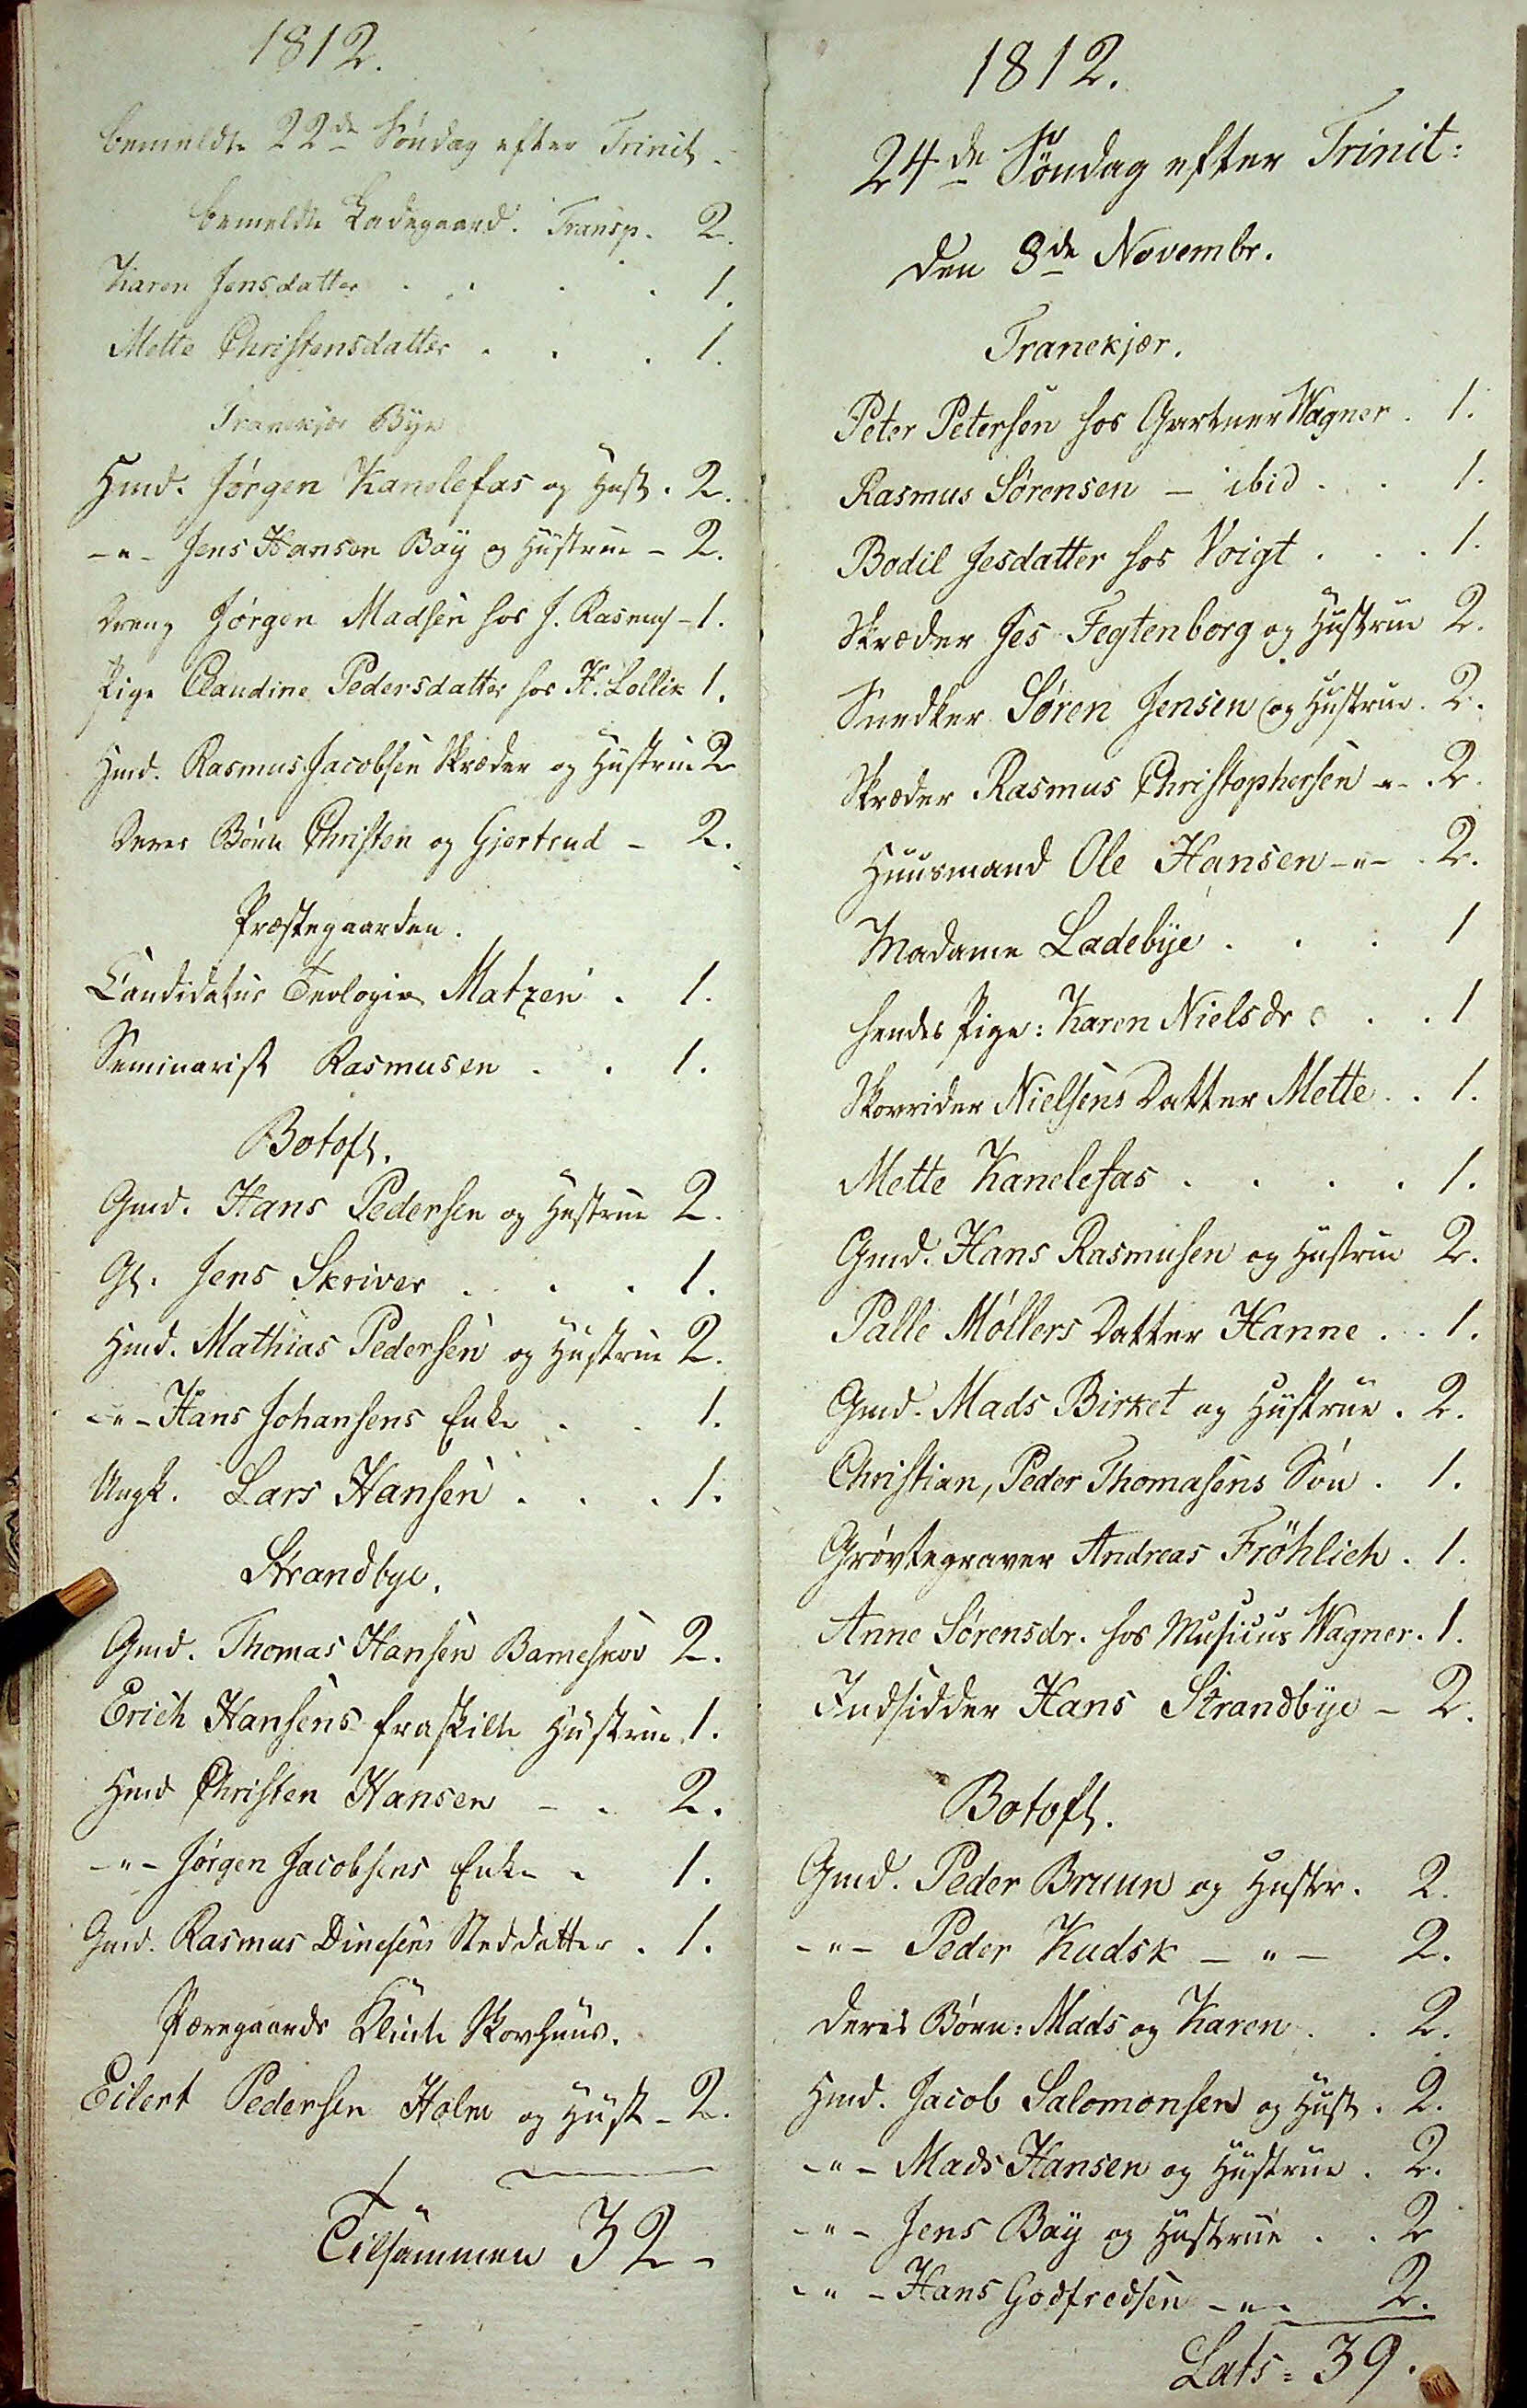1812
bemeldte 22de Søndag efter Trinit.
bemeldte Ladegaard. Transp. 2.
Karen JensDatter . . . . 1.
Mette Christensdatter . . . 1.
Tranekjær Bye
Hmd. Jørgen Kanelefas og Hust . 2.
-"- Jens Hansøn Bay og Hustrue - 2.
Dreng Jørgen Madsen hos J Rasmus - 1.
Pige Claudine Pedersdatter hos H. Lollik 1.
Hmd. Rasmus Jacobsen Skræder og Hustrue 2
deres Børn Christen og Gjertrud - 2.
Præstegaarden.
Candidatus Teologie## Matzen . 1.
Seminarist Rasmusen . . 1.
Botoft.
Gmd. Hans Pedersen og Hustrue 2.
Gl. Jens Skriver . . . 1.
Hmd. Mathias Pedersen og Hustrue 2.
-"- Hans Johansens Enke . . 1.
Ungk. Lars Hansen . . . 1.
Strandbye.
Gmd. Thomas Hansen Bameskov 2.
Erich Hansens fraskilt Hustrue . 1.
Hmd Christen Hansen - . 2.
-"- Jørgen Jacobsens Enke . 1.
Gmd. Rasmus Dinesens Steddatter . 1.
Pæregaards Klint Skovhuus.
Eilert Pedersen Holm og Hust - 2.
Tilsammen 32 -
1812.
24de Søndag efter Trinit:
den 8de Novembr.
Tranekjær.
Peter Petersen hos Gartner Wagner . 1.
Rasmus Sørensen - ibid . . 1.
Bodil Jesdatter hos Voigt . . . 1
Skræder Jes Fegtenborg og Hustrue 2.
Snedker Søren Jensen og Hustrue . 2.
Skræder Rasmus Christophersen -"- . 2.
Huusmand Ole Hansen -"- . 2.
Madame Ladebye . . . 1
hendes Pige: Karen Nielsdr . . . 1
Skovrider Nielsens Datter Mette . . 1.
Mette Kanelefas . . . . 1.
Gmd. Hans Rasmusen og Hustrue 2.
Palle Møllers Datter Hanne . . 1.
Gmd. Mads Birket og Hustrue . 2.
Christian, Peder Thomasens Søn . 1.
Grøvtegraver Andreas Frøhlich . 1.
Anne Sørensdr. hos Musicus Wagner . 1.
Indsidder Hans Strandbye - 2.
Botoft.
Gmd. Peder Bruun og Hustr . 2.
-"- Peder Kudsk  -"- 2.
deres Børn: Mads og Karen . . 2.
Hmd. Jacob Salomonsen og Hust . 2.
-"- Mads Hansen og Hustrue . 2.
-"- Jens Bay og Hustrue . . 2
-"- Hans Godfredsen -"- 2.
Lats = 39.
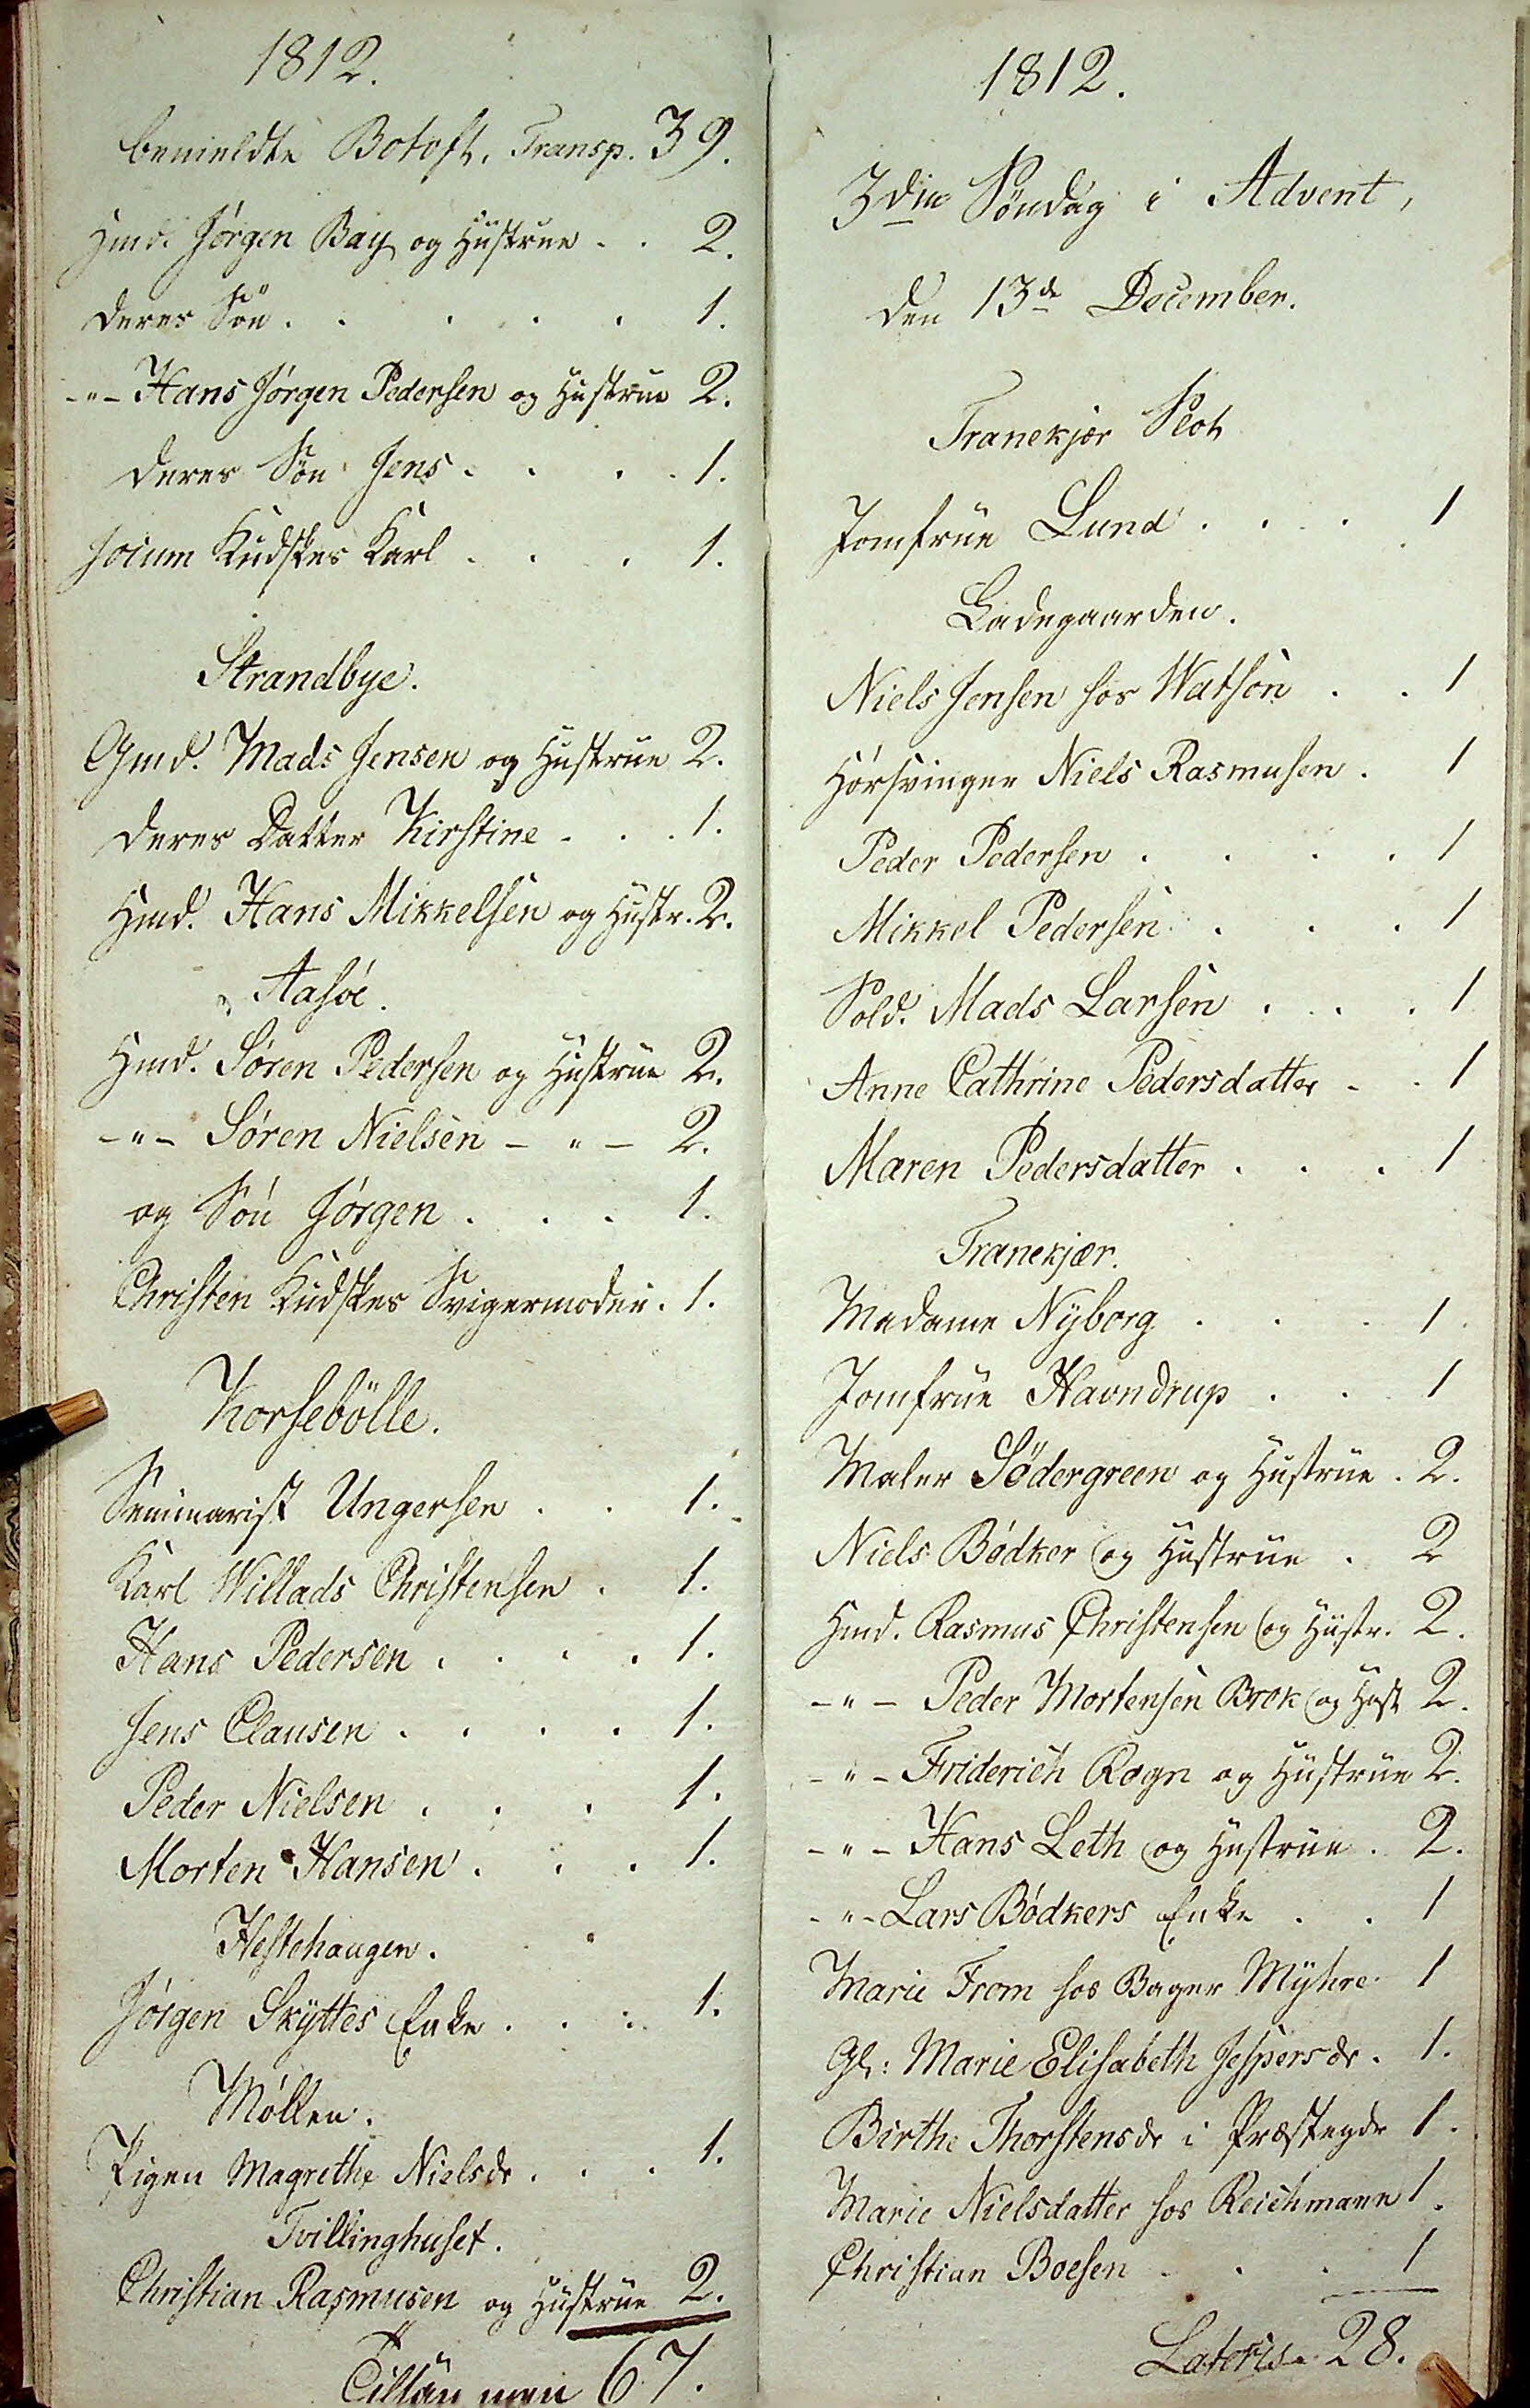1812
bemeldte Botoft. Transp. 39.
Hmd. Jørgen Bay og Hustrue . . 2.
deres Søn . . . . . 1.
-"- Hans Jørgen Pedersen og Hustrue 2.
deres Søn Jens . . . . 1.
Jocum Kudskes Karl . . . 1.
Strandbye.
Gmd. Mads Jensen og Hustrue 2.
deres Datter Kirstine . . . 1.
Hmd. Hans Mikkelsen og Hustr . 2.
Aasøe
Hmd. Søren Pedersen og Hustrue 2.
-''- Søren Nielsen -"- 2.
og Søn Jørgen . . . 1.
Christen Kudskes Svigermoder . 1.
Korsebølle.
Seminarist Ungersen . . 1.
Karl Willads Christensen . 1.
Hans Pedersen . . . . 1.
Jens Clausen . . . . 1.
Peder Nielsen . . . 1.
Morten Hansen. . . 1.
Hestehaugen.
Jørgen Skyttes Enke . . . 1.
Møllen.
Pigen Magrethe Nielsdr . . . 1.
Tvillinghuset.
Christian Rasmusen og Hustrue 2.
Tilsammen 67
1812.
3die Søndag i Advent,
den 13de December.
Tranekjær Slot
Jomfrue Lund . . . 1
Ladegaarden.
Niels Jensen hos Watson . . 1
Hørsvinger Niels Rasmusen . 1
Peder Pedersen . . . . 1
Mikkel Pedersen . . . 1
Sold. Mads Larsen . . . 1
Anne Cathrine Pedersdatter . . 1
Maren Pedersdatter . . . 1
Tranekjær.
Madame Nyborg . . . 1
Jomfrue Havndrup . . 1
Maler Sødergreen og Hustrue . 2.
Niels Bødker og Hustrue . 2
Hmd. Rasmus Christensen og Hustr . 2.
-"- Peder Mortensen Brok og Hust 2.
-"- Friderich Rogn og Hustrue 2.
-"- Hans Leth og Hustrue . 2.
-"- Lars Bødkers Enke . . 1
Marie From hos Bager Myhre . 1
Gl: Marie Elisabeth Jespersdr . 1.
Birthe Thorstensdr i Præstegdr 1.
Marie Nielsdatter hos Reichmann 1.
Christian Boesen . . . 1
Lateris. 28.